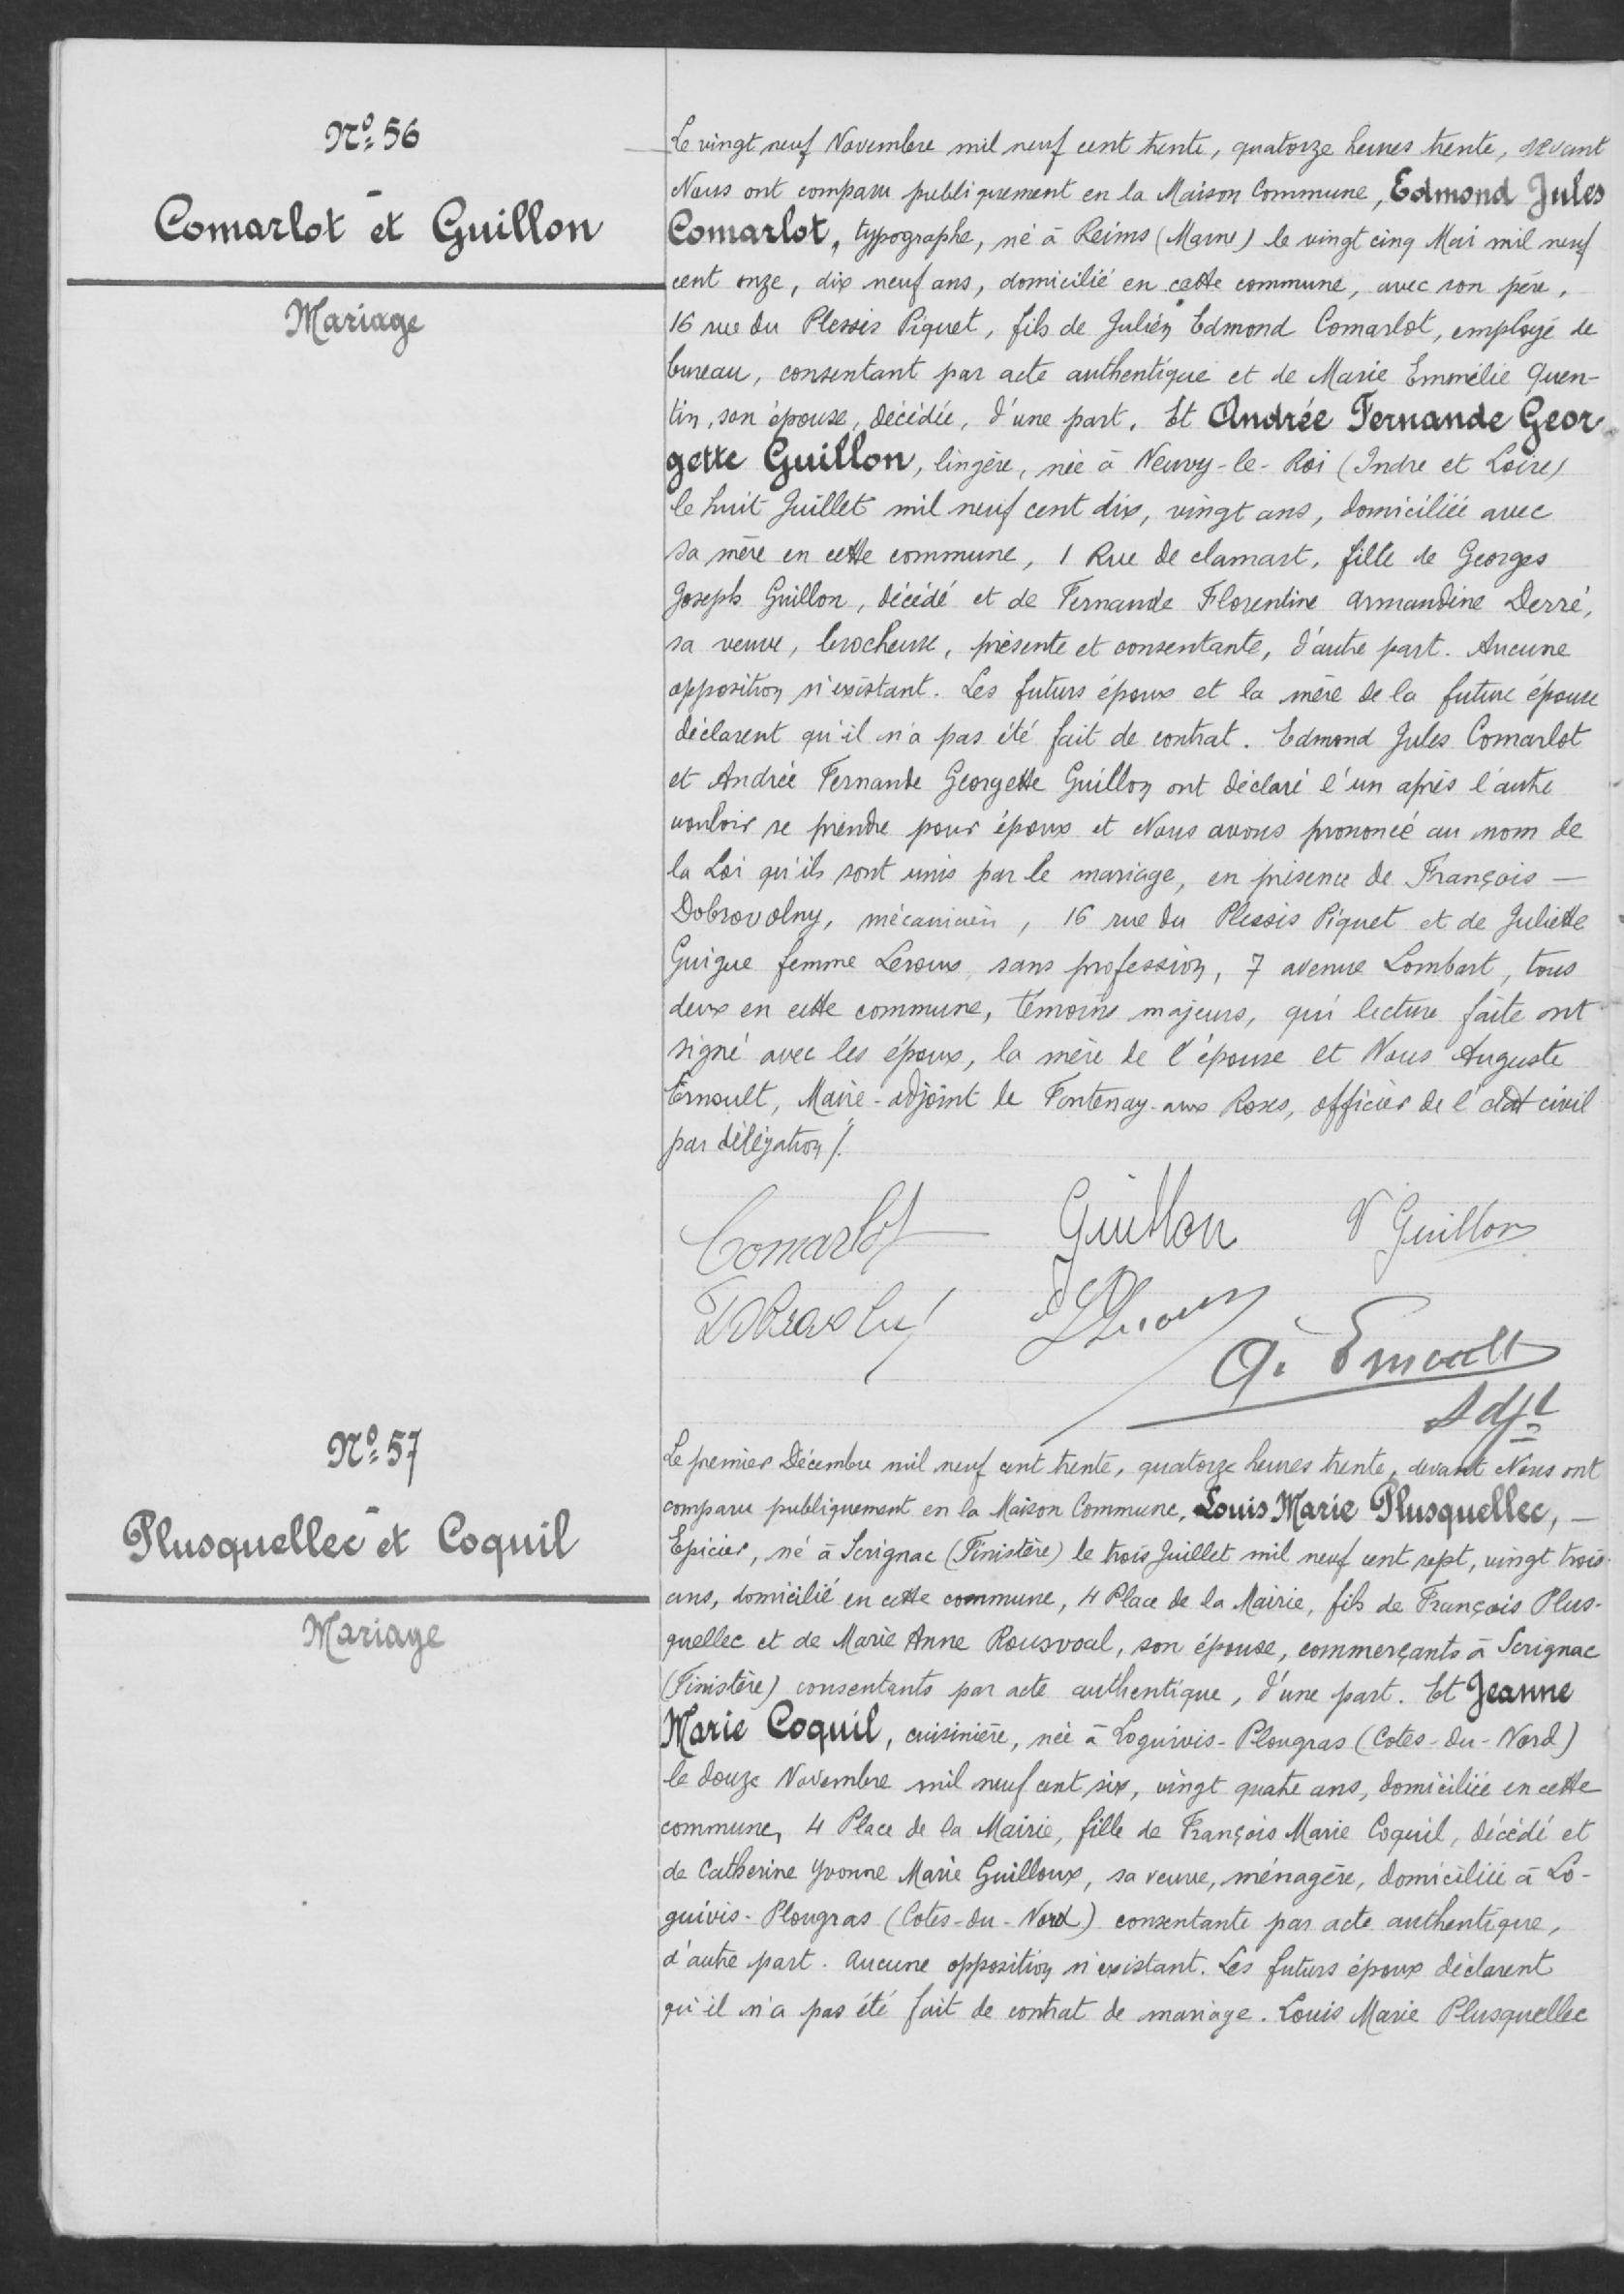N°56
Comarlot et Guillon
Mariage
Le vingt neuf Novembre mil neuf cent trente, quatorze heures trente, devant
nous ont comparu publiquement en la Maison Commune, Edmonde Jules
Comarlot, typographe, né à Reims (Marne) le vingt cinq Mai mil neuf
cent onze, dix neuf ans, domicilié en casse commune, avec son père,
16 rue du Plessi Piquet, fils de Julien Edmond Comarlot, employé de
bureau, consentant par acte authentique et de Marie Emmélie Quen-
tin, son épouse, décédée, d'un part. Et Andrée Fernande Geor
gette Guillon, lingère, né à Neuvy-le-Roi (Indre et Loire)
le huit Juillet mil neuf cent dix, vingt ans, domiciliée avec
sa mère en cette commune, 1 Rue de clamart, fille de Georges
Joseph Guillon, décédé et de Fernande Florentin armandine Derré
sa veuve, brocheuse, présente et consentante, d'autre part. Aucune
opposition n'existant. Les futurs époux et la mère de la future épouse
déclarent qu'il n'a pas été fait de contrat. Edmond Jules Comarlot
et Andrée Fernande Georgette Guillon ont déclaré l'un après l'autre
vouloir se prendre pour époux et Nous avons prononcé au nom de
la Loi qu'ils sont unis par le mariage, en présence de François-
Dobrovolny, mécanicien, 16 rue de la Plesis Piquet et de Juliette
Guigue femme Leroin, sans profession, 7 avenue Lombart, tous
deux en cette commune, témoins majeurs, qui lecture faite ont
signé avec les époux, la mère de l'épouse et Nous Auguste
Ernoult, Maire-adjoint de Fontenay-aux-Roses, officier de l'état civil
par délégation./.
N° 57
Plusquellec et Coquil
Mariage
Le premier décembre mil neuf cent trente, quatorze heures trente devant Nous ont
comparu publiquement en la Maison Commune, Louis Marie Pluequellec, -
Epicier, né à Sevignac (Finistère) le trois Juillet mil neuf cent sept, vingt trois
ans, domicilié en cette commune, 4 Place de la Mairie, fils de François Plus-
gnellec et de Marie Anne Rousvoal, son épouse, commerçants à Serignac
(Finistère) consentants par acte authentique, d'une part. Et Jeanne
Marie Coquil, cuisinière, née à Loguivis-Plougras (Côtes-du-Nord)
le douze Novembre mil neuf cent six, vingt quatre ans, domiciliée en cette
commune, 4 Place de la Mairie, fille de François Marie Coquil, décédé et
de Catherine Yvonne Marie Guilloux, sa veuve, ménagère, domiciliée à Lo-
guivis-Ploudras (Cotes-du-Nord) consentante par acte authentique,
d'autre part. aucune opposition n'existant. Les futurs époux déclarent
qu'il n'a pas été fait de contrat de mariage. Louis Marie Plusquellec
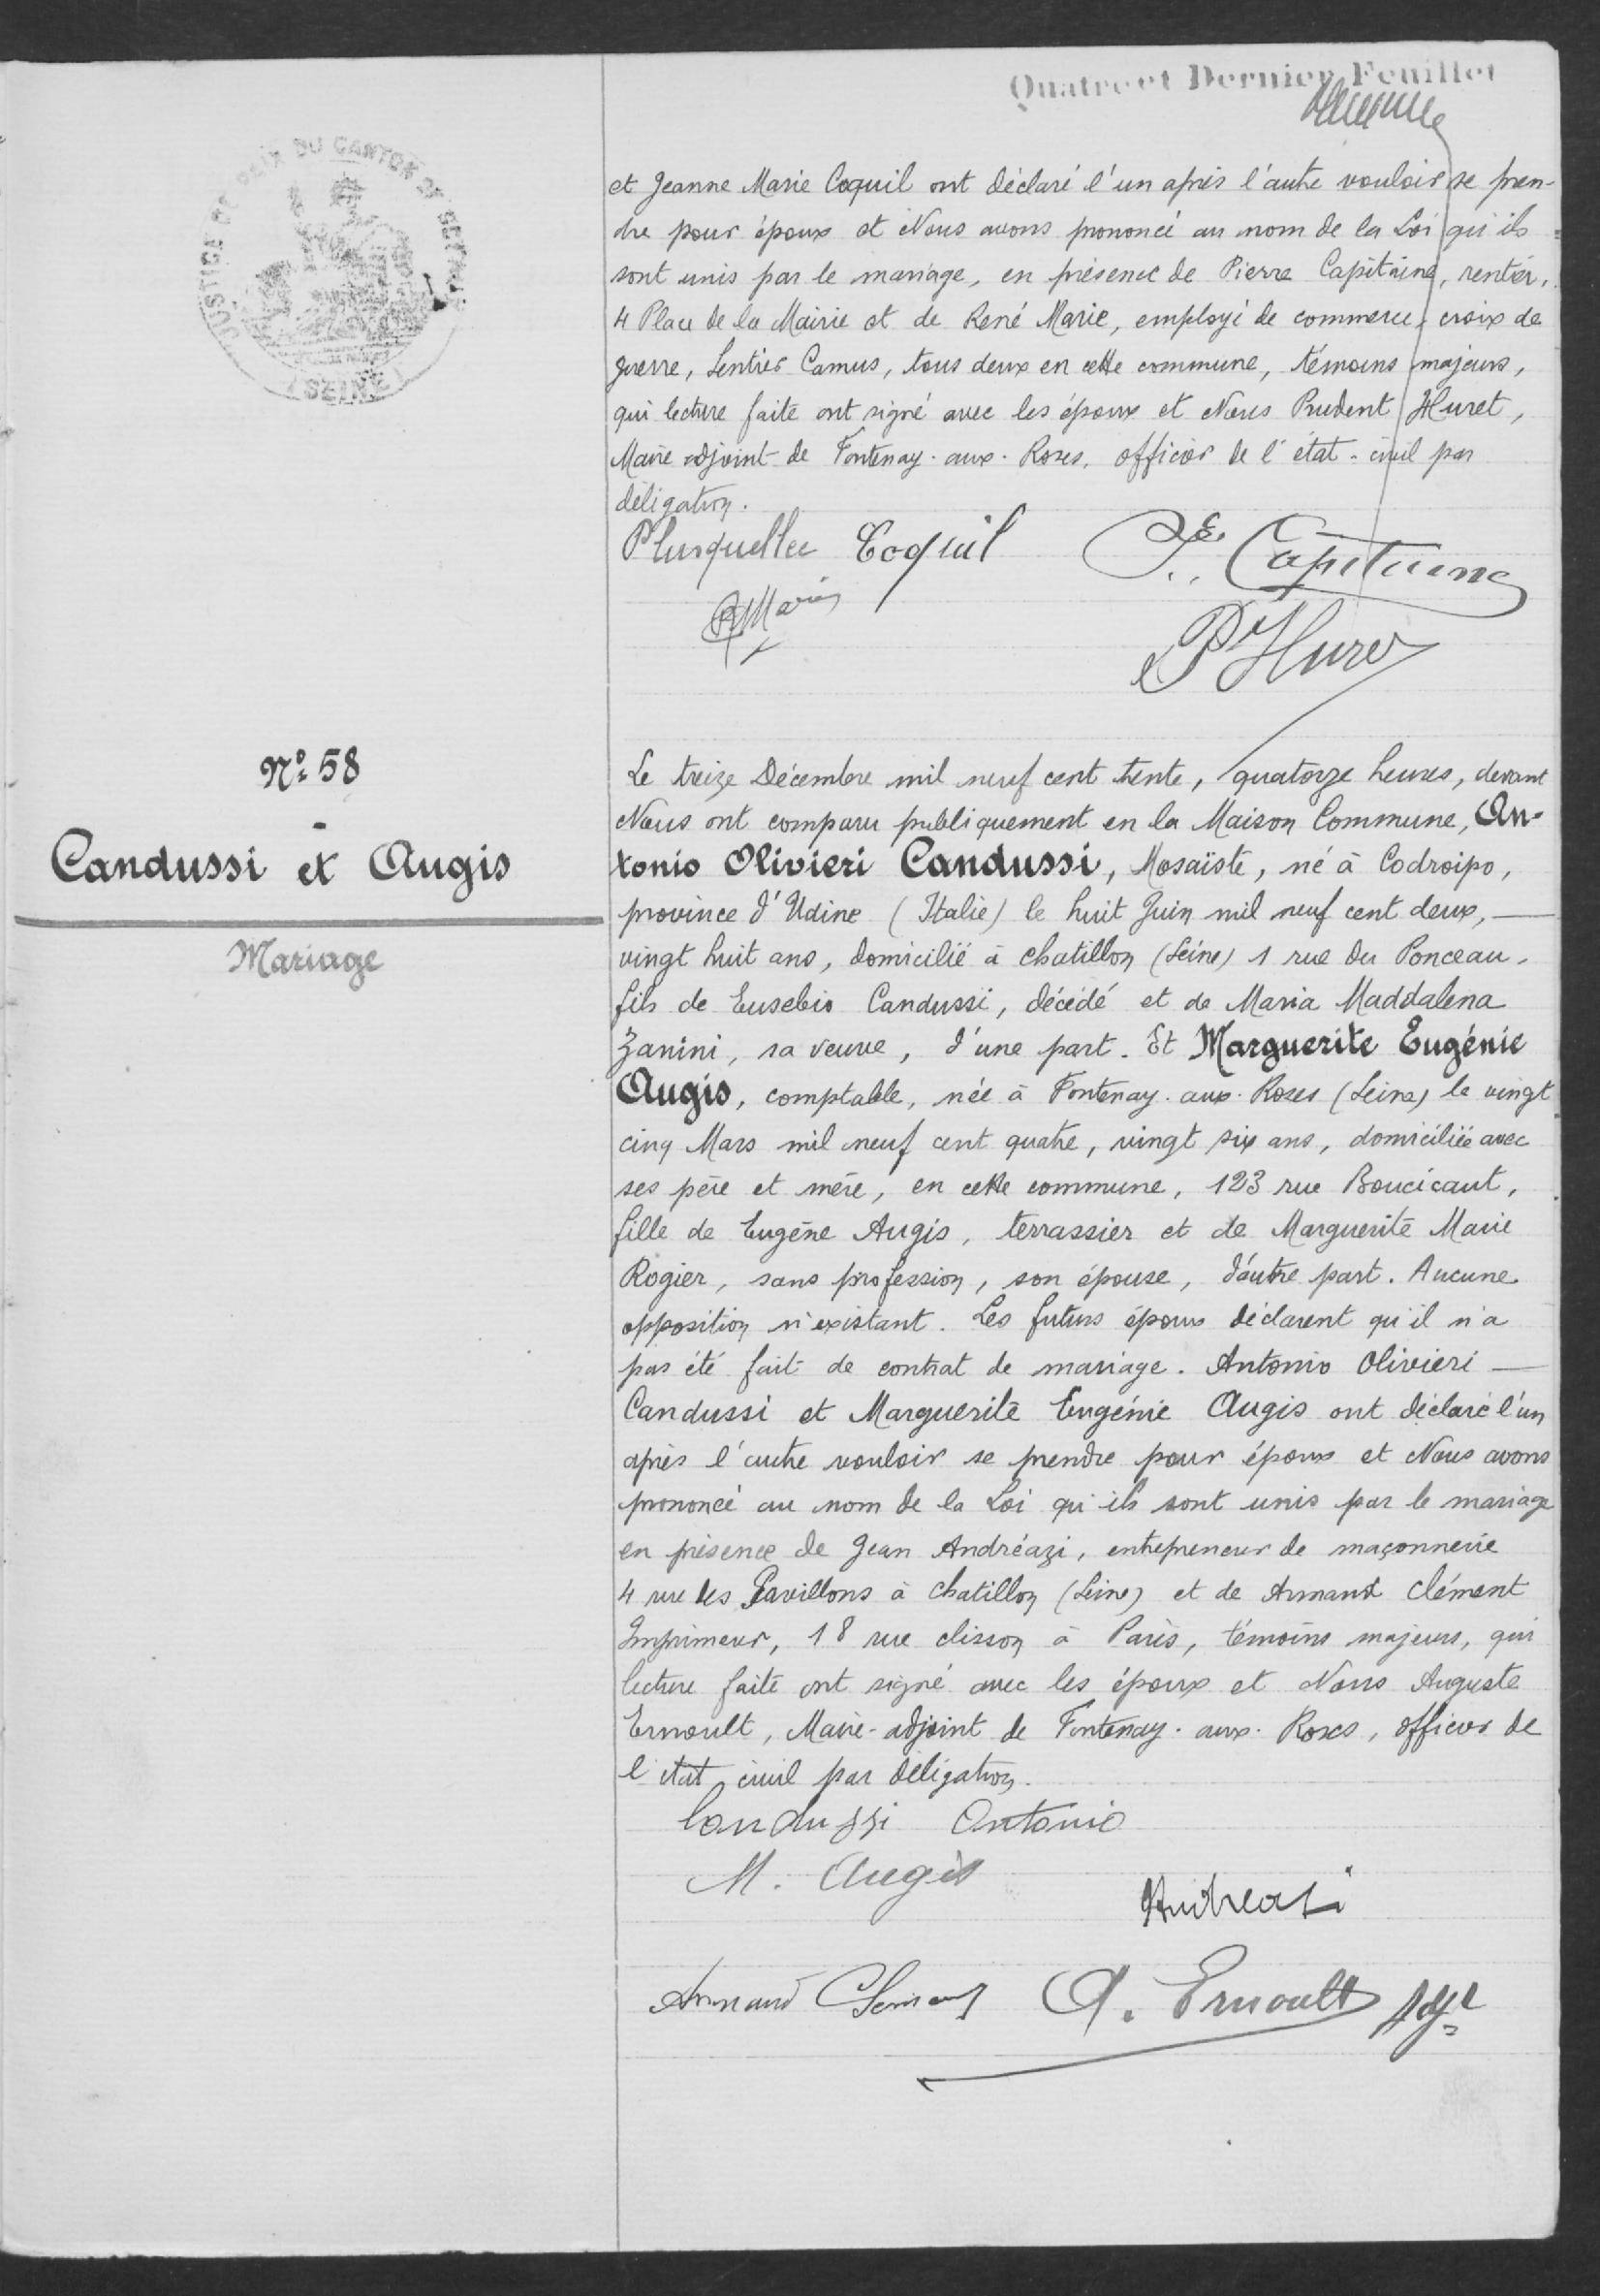et Heanne Marie Coquil ont déclaré l'un après l'autre vouloir se pren-
dre pour époux et Nous avons prononcé au nom de la Loi qu'ils
sont unis par le mariage, en présence de Pierre Capitaine, rentier,
4 Place de la Mairie et de René Marie, employé de commerce, croix de
guerre, Sentier Camus, tous deux en cette commune, témoins majeurs,
qui lecture faite ont signé avec les époux et Nous Prudent Huret,
Maire adjoint de Fontenay-aux-Roses, officier de l'état-civil par
délégation.
N°58
Candussi et Augis
Mariage
Le treize Décembre mil neuf cent trente, quatorze heures, devant
Nous ont comparu publiquement en la Maison Commune, An-
xonis Olivieri Candussi, Mosaïste, né à Codroipo
province d'Udine (Italie) le huit juin mil neuf cent deux, -
vingt huit ans, domicilié à Chatillon (Seine) 1 rue du Ponceau,
fils de Eusebis Candussi, décédé et de Maria Maddalena
Zanini, sa veuve, d'une part. Et Marguerite Eugénie
Augis, comptable, née à Fontenay-aux-Roses (Seine) le vingt
cinq Mars mil neuf cent quatre, vingt six ans, domiciliée avec
ses père et mère, en cette commun, 123 rue Boucicaut,
fille de Eugène Augis, terrassier et de Marguerite Marie
Rogier, sans profession, son épouse, d'autre part. Aucune
opposition n'existant. Les futurs époux déclarent qu'il n'a
pas été fait de contrat de mariage. Antonio Olivieri-
Condussi et Marguerite Eugénie Augis ont déclaré l'un
après l'autre vouloir se prendre pour époux et Nous avons
prononcé au nom de la Loi qu'ils sont unis par le mariage
en présence de Jean Andréazi, entrepreneur de maçonnerie
4 rue les Pavillons à chatillon (Seine) et de Armant Clément
Imprimeur, 18 rue clisson à Paris, témoins majeurs, qui
lecture faite ont signé avec les époux et Nous Auguste
Ernoult, Marie adjoint de Fontenay-aux-Roses, officier de
l'état civil par délégation.
Candussi Antonio
M. Augis